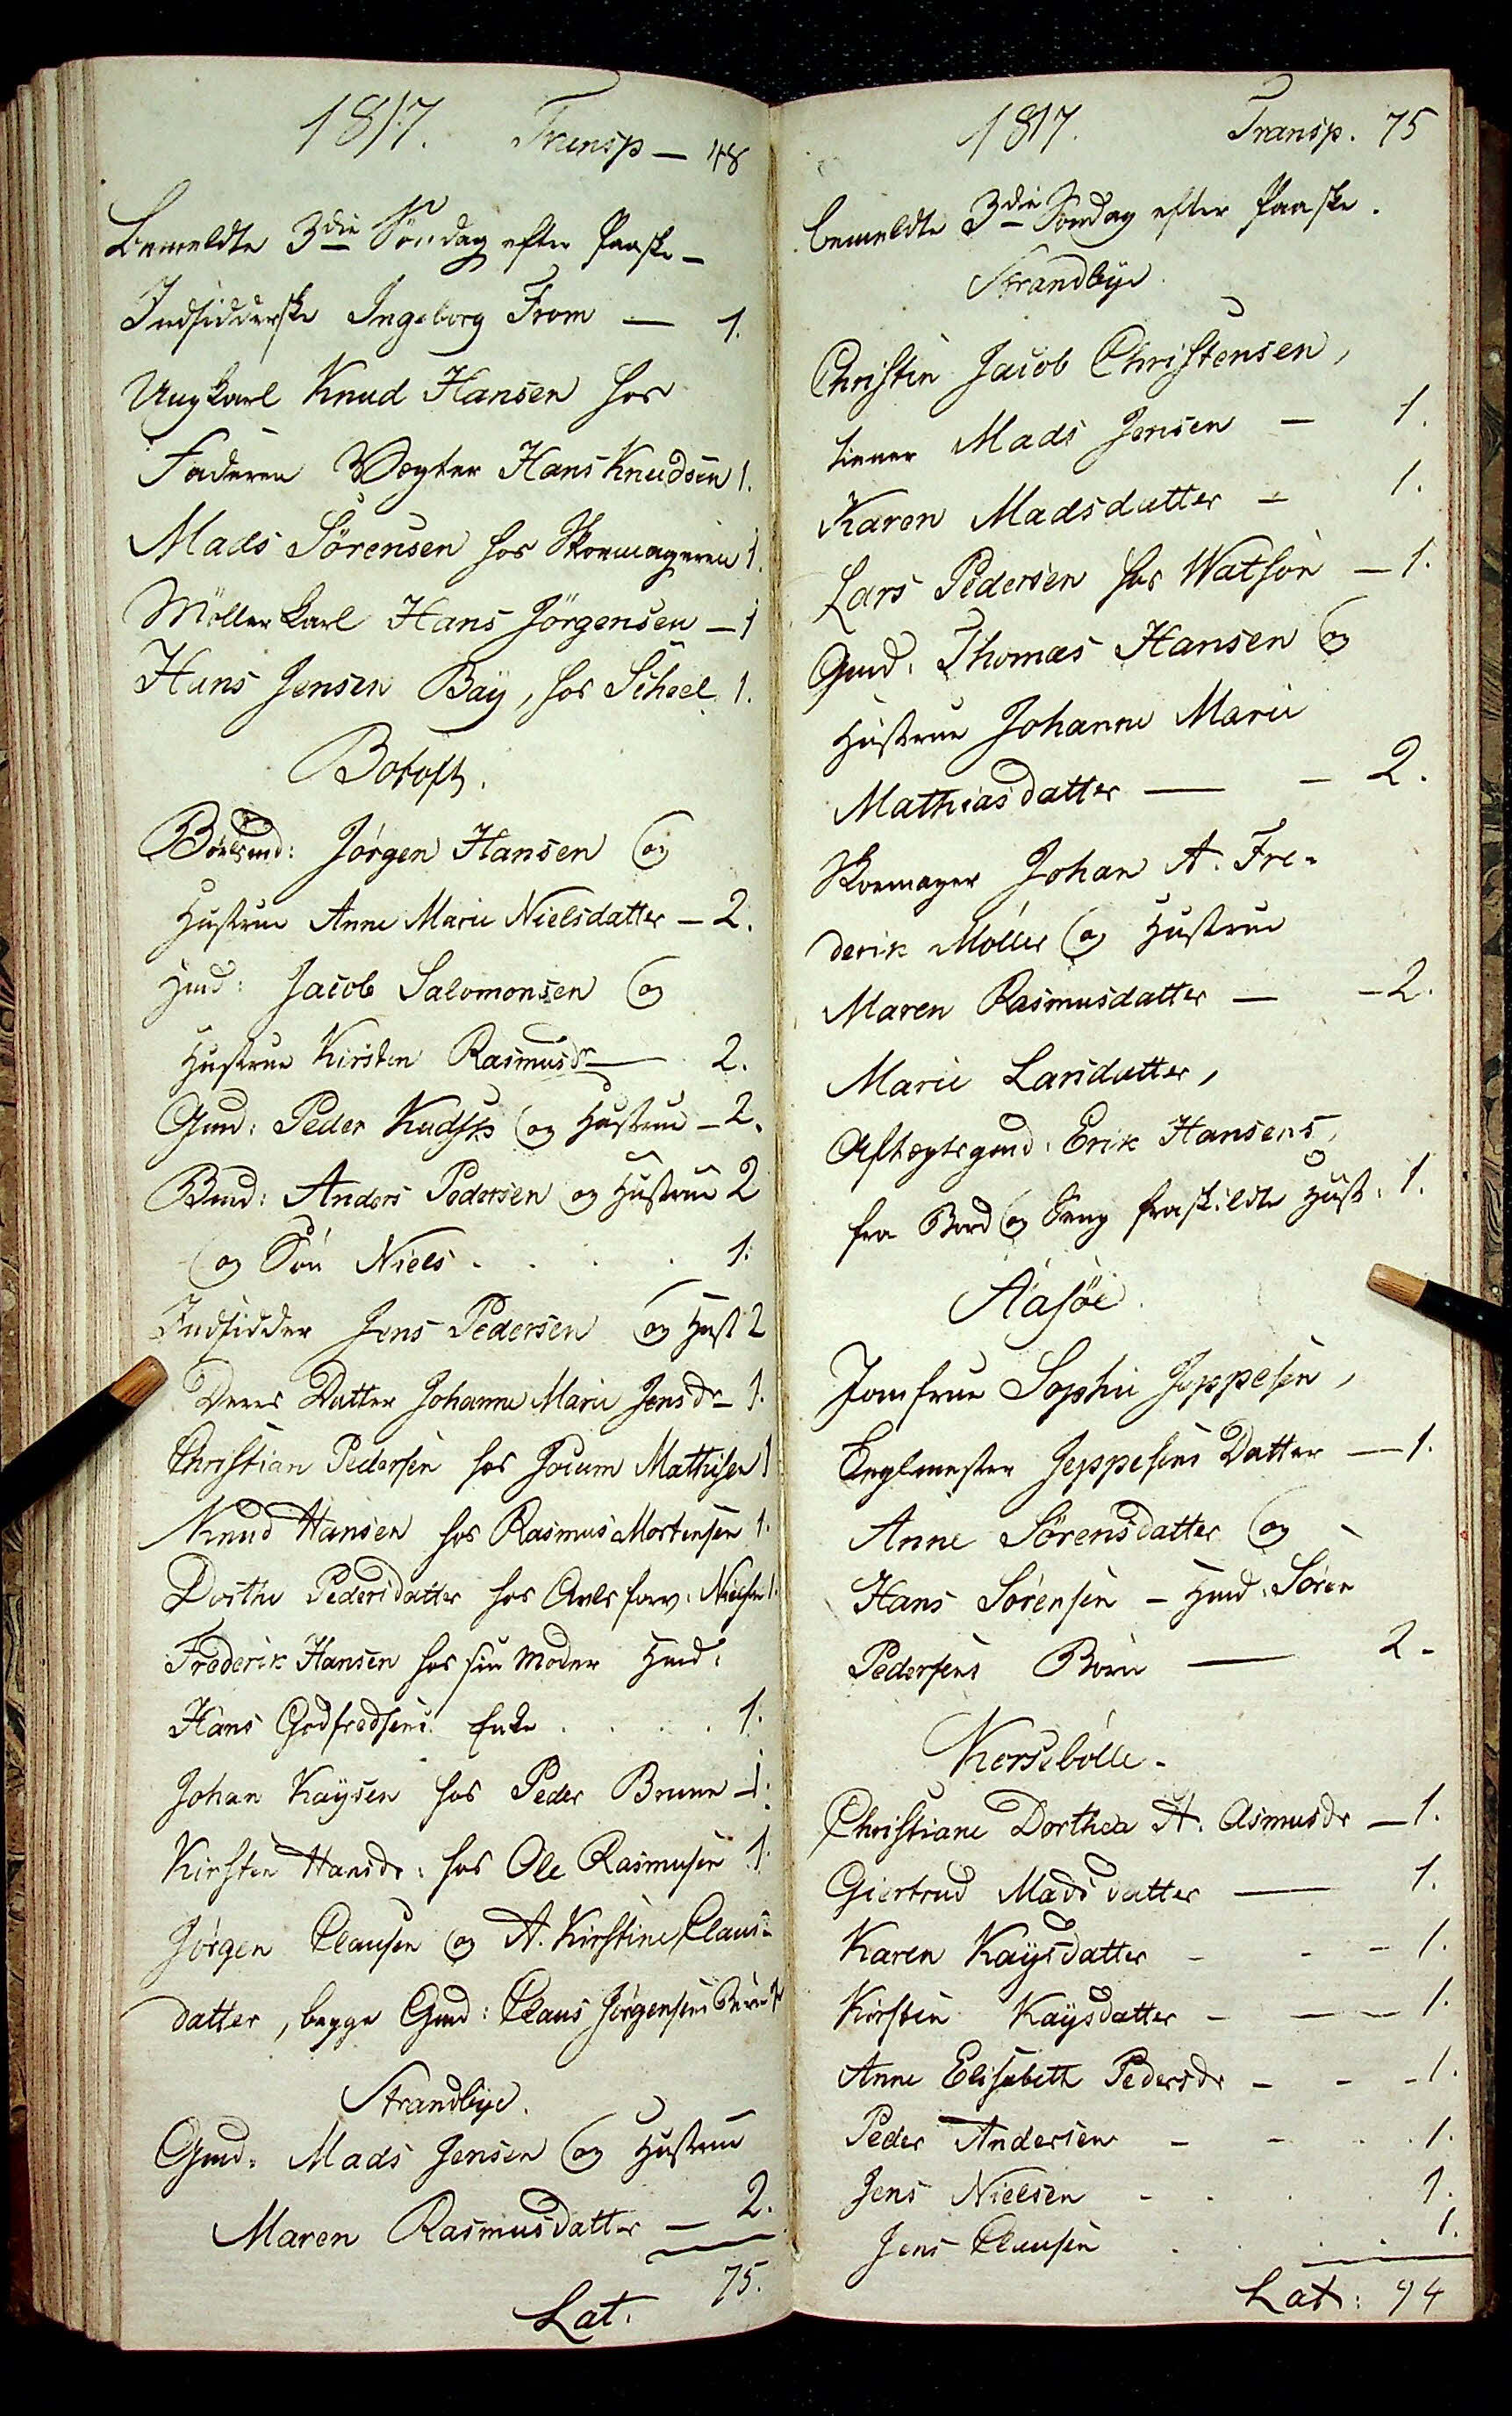1817.
Transp - 48
bemeldte 3die Søndag efter Paaske-
Indsidderske Ingeborg From -1.
Ungkarl Knud Hansen hos
Faderen Vægter Hans Knudsen 1.
Mads Sørensen hos Skoemageren 1.
Møllerkarl Hans Jørgensen - 1
Hans Jensen Bag, hos Scheel 1.
Botoft
Boelsmd: Jørgen Hansen og
Hustrue Anne Marie Nielsdatter - 2.
Hmd: Jacob Salomonsen og
Hustrue Kirsten Rasmusdr - 2.
Gmd: Peder Kudsk og Hustrue - 2.
Bmd: Anders Pedersen og Hustrue 2
og Søn Niels . . . .  1.
Indsidder Jens Pedersen og Hust 2
Deres Datter Johanne Marie Jensdr -1.
Christian Pedersen hos Jocum Mathisen 1
Knud Hansen hos Rasmus Mortensen 1.
Dorthe Pedersdatter hos Avlsforv: Nielsen 1.
Friderik Hansen hos sin Moder Hmd:
Hans Godfredsens Enke . . . . 1.
Johan Kaysen hos Peder Bruun - 1.
Kirsten Hansdr: hos Ole Rasmusen 1.
Jørgen Clausen og A. Kirstine Claus¬
datter, begge Gmd: Claus Jørgensens Børn 2
Strandbye.
Grd: Mads Jensen og Hustrue
Maren Rasmusdatter - 2.
Lat. 75
1817
Transp. 75
bemeldte 3die Søndag efter Paaske.
Strandbye
Christen Jacob Christensen,
tiener Mads Jensen - 1.
Karen Madsdatter - 1.
Lars Pedersen hos Watson - 1.
Gmd: Thomas Hansen og
Hustrue Johanne Marie
Mathiasdatter - - 2.
Skoemager Johan A. Fre¬
derik Møller og Hustrue
Maren Rasmusdatter - - 2
Marie Larsdatter,
Aftægtsgmd: Erik Hansens
fra Bord og Seng fraskildte Hust: 1.
Aasøe
Jomfrue Sophie Jeppesen,
Teglmester Jeppesens Datter - 1.
Anne Sørensdatter og
Hans Sørensen - Hmd: Søren
Pedersens Børn - 2-
Korsebølle.
Christiane Dorthea H. Asmusdr - 1.
Giertrud Madsdatter - 1.
Karen Kaysdatter - - - 1.
Kirsten Kaysdatter - - - 1.
Anne Elisabeth Pedersdr - - - 1.
Peder Andersen - - . . 1.
Jens Nielsen . . . . 1.
Jens Clausen . . . . 1.
Lat: 94
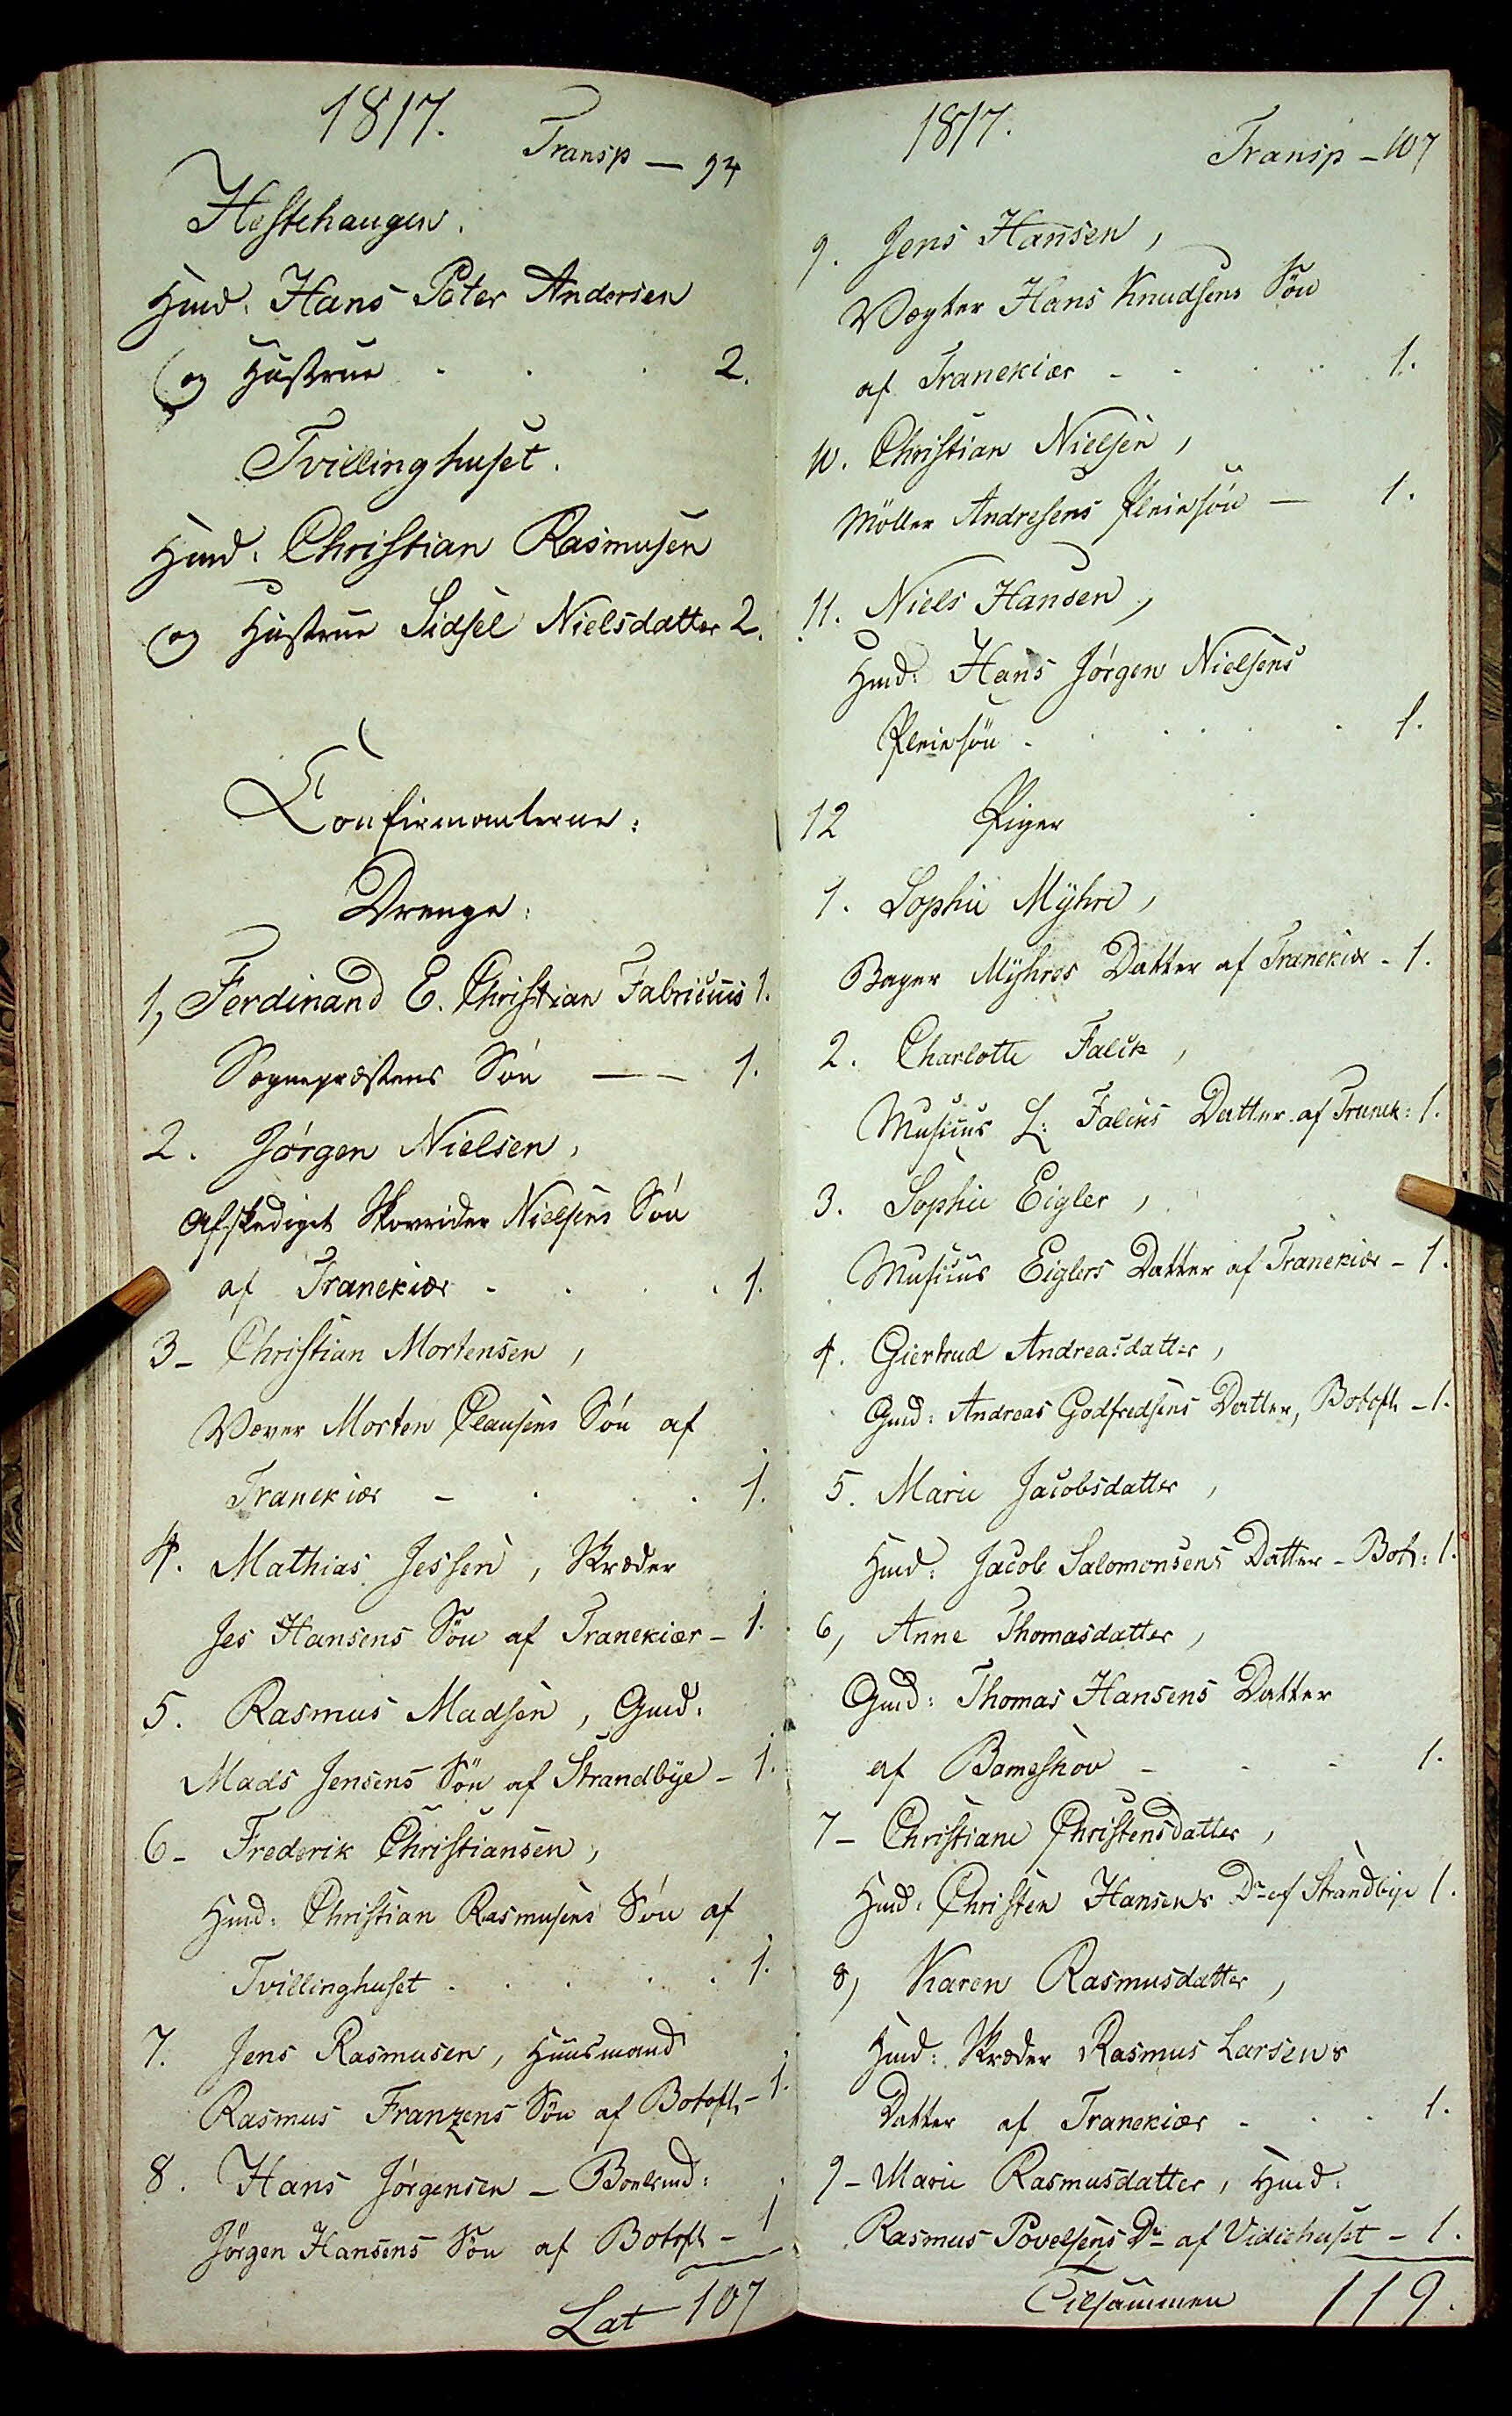1817.
Transp - 94
Hestehaugen.
Hmd, Hans Peder Andersen
og Hustrue . . . 2.
Tvillinghuset.
Hmd: Christian Rasmusen
og Hustrue Sidsel Nielsdatter 2.
Confirmanterne:
Drenge:
1 Ferdinand E. Christian Fabricius 1.
Sognepræstens Søn - - 1.
2. Jørgen Nielsen,
Afskediget Skovrider Nielsens Søn
af Tranekiær . . . 1.
3. Christian Mortensen
Væver Morten Clausens Søn af
Tranekiær - . . . 1.
4. Mathias Jessen, Skræder
Jes Hansens Søn af Tranekiær - 1.
5. Rasmus Madsen, Gmd:
Mads Jensens Søn af Strandbye - 1.
6. Frederik Christiansen,
Hmd: Christian Rasmusens Søn af
Tvillinghuset . . . . . 1.
7. Jens Rasmusen, Huusmand
Rasmus Franzens Søn af Botoft -1.
8. Hans Jørgensen - Boelsmd:
Jørgen Hansens Søn af Botoft -1
Lat 167
1817
Transp - 107
9. Jens Hansen,
Vægter Hans Knudsens Søn
af Tranekiær . . . .1.
10. Christian Nielsen,
Møller Andresens Pleiesøn - 1.
11. Niels Hansen,
Hmd: Hans Jørgen Nielsens
Pleiesøn . . . . . . 1.
12 Piger
1. Sophie Myhre,
Bager Myhres Datter af Tranekiær . 1.
2. Charlotte Falck
Musicus L: Falcks Datter af Tranek: 1.
3. Sophie Eigler
Musicus Eiglers Datter af Tranekiær - 1.
4. Giertrud Andreasdatter,
Gmd: Andreas Godfredsens Datter, Botoft - 1.
5 Marie Jacobsdatter
Hmd: Jacob Salomonsens Datter Bot: 1
6, Anne Thomasdatter
Gmd: Thomas Hansens Datter
af Bameskov - - - 1.
7 - Christiane Christensdatter
Hmd: Christen Hansens Dr af Strandbye 1
8, Karen Rasmusdatter
Hmd: Skræder Rasmus Larsens
Datter af Tranekiærm. . . 1.
9 - Marie Rasmusdatter, Hmd:
Rasmus Povelsens Dr af Vidiehuset - 1
Tilsammen 119